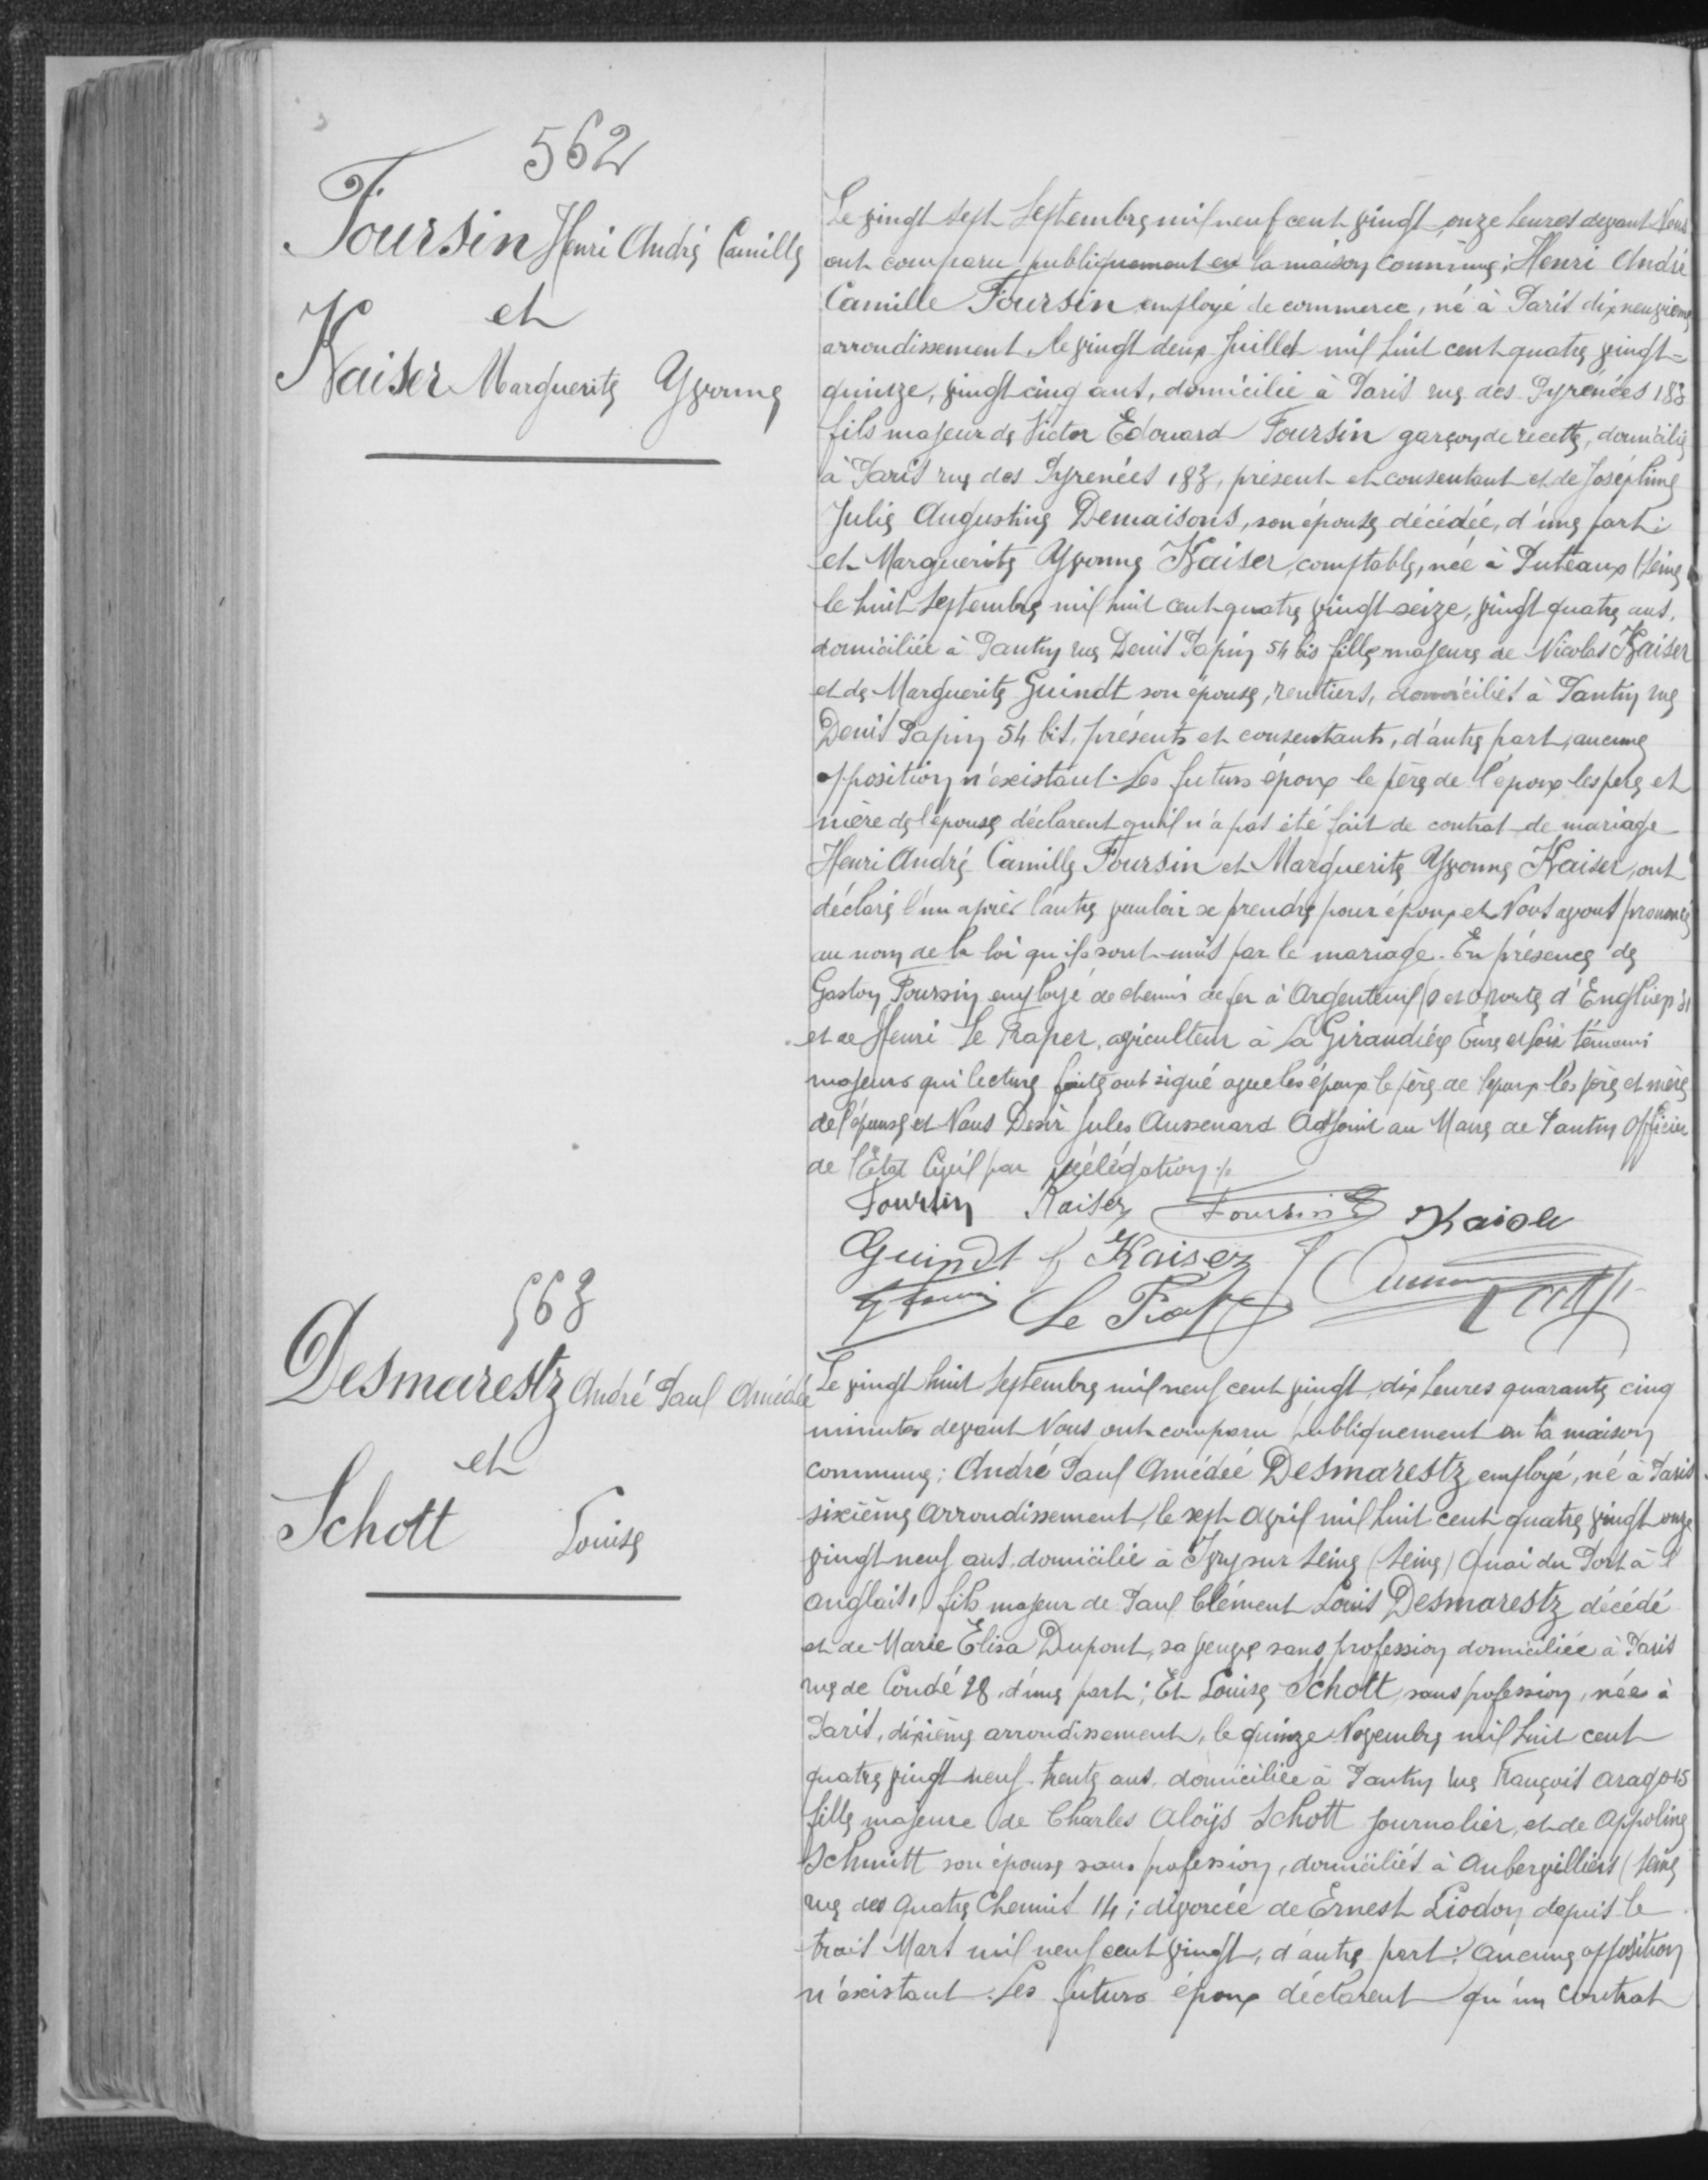562
Foursin Henri André Camille
et
Kaiser Marguerite Yvonne
Le vingt sept Septembre mil neuf cent vingt onze heure devant Nous
ont comparu publiquement en la maison commune; Henri André
Camille Foursin employé de commerce, né à Paris dix neuvième
arrondissement, le vingt deux Juillet mil huit cent quatre vingt-
quinze, vingt cinq ans, domiciliée à Paris rue de Pyrénées 183
fils majeur de Victor Edouard Foursin garçon de recette domicilié
à Paris rue des Pyrenées 183, présent et consentant, et de Joséphine
Julie Augustine Demaisons, son épouse, décédée, d'une part.
et Marguerite Yvonne Kaiser, comptable, née à Puteaux (Seine
le huit Septembre mil huit cent-quatre vingt-seize, vingt quatre ans,
domiciliée à Pantin rue Denis Papin, 54 bis fille majeure de Nicolas Kaiser
et de Marguerite Guindt son épouse, rentiers, domiciliés à Pantin rue
Denis Papin, 54 bis présents et consentants, d'autre part, aucune
opposition n'existant. Les futurs époux le père de l'époux les pere et
mère de l'épouse déclarent qu'il n'a pas été fait de contrat de mariage
Henri André Camille Foursin et Marguerite Yvonne Kaiser, ont
déclaré l'un après l'autre vouloir se prendre pour époux et Nous avons prononcé
au nom de la loi qu'ils sont unis par le mariage. En présence de
Gaston Foursin employé de chemin de fer à Argenteuil (S et O) route d'Englien 31
et de Henri le Frapier, agriculteur à la Giraudière Eure et Loir témoins
majeurs qui lecture faite ont signé avec les époux, le père de l'époux, les père et mère
de l'épouse et Nous Desir Jules Aussenard Adjoit au Maire de Pantin Officier
de l'Etat Civil par délégation ./.
563
Desmarestz André Paul Amédée
et
Schott Louise
Le vingt huit septembre mil neuf cent vingt dix heures quanrante cinq
minutes devant Nous ont comparu publiquement en la maison,
commune: André Paul Amédée Desmarestz, employé, né à Paris
sixième Arrondissement, le sept Avril mil huit cent quatre vingt onze
vingt-neuf ans domicilié à Issy sur Seine (Seine) Quai du Port à l'
Anglais, fils majeur de Paul Clément Louis Desmarestz décédé
et de Marie Elisa Dupont, sa veuve sans profession, domiciliée à Paris
rue de Condé 28, d'une part; Et Louise Schott, sans profession, née à
Paris, dixième arrondissement, le quinze Novembre mil huit cent
quatre vingt neuf, trente ans, domicilié à Pantin rue François Arago 15
fille majeure de Charles Aloys Schott journalier et de Appoline
Schmitt son épouse, sans profession, domiciliés à Aubervilliers (Seine
rue des Quatre Chemins, 14; divorcée de Ernest Liodoy, depuis le
trois Mars mil neuf cent vingt, d'autre part: Aucune opposition
n'existant. Les futurs époux déclarent qu'un contrat
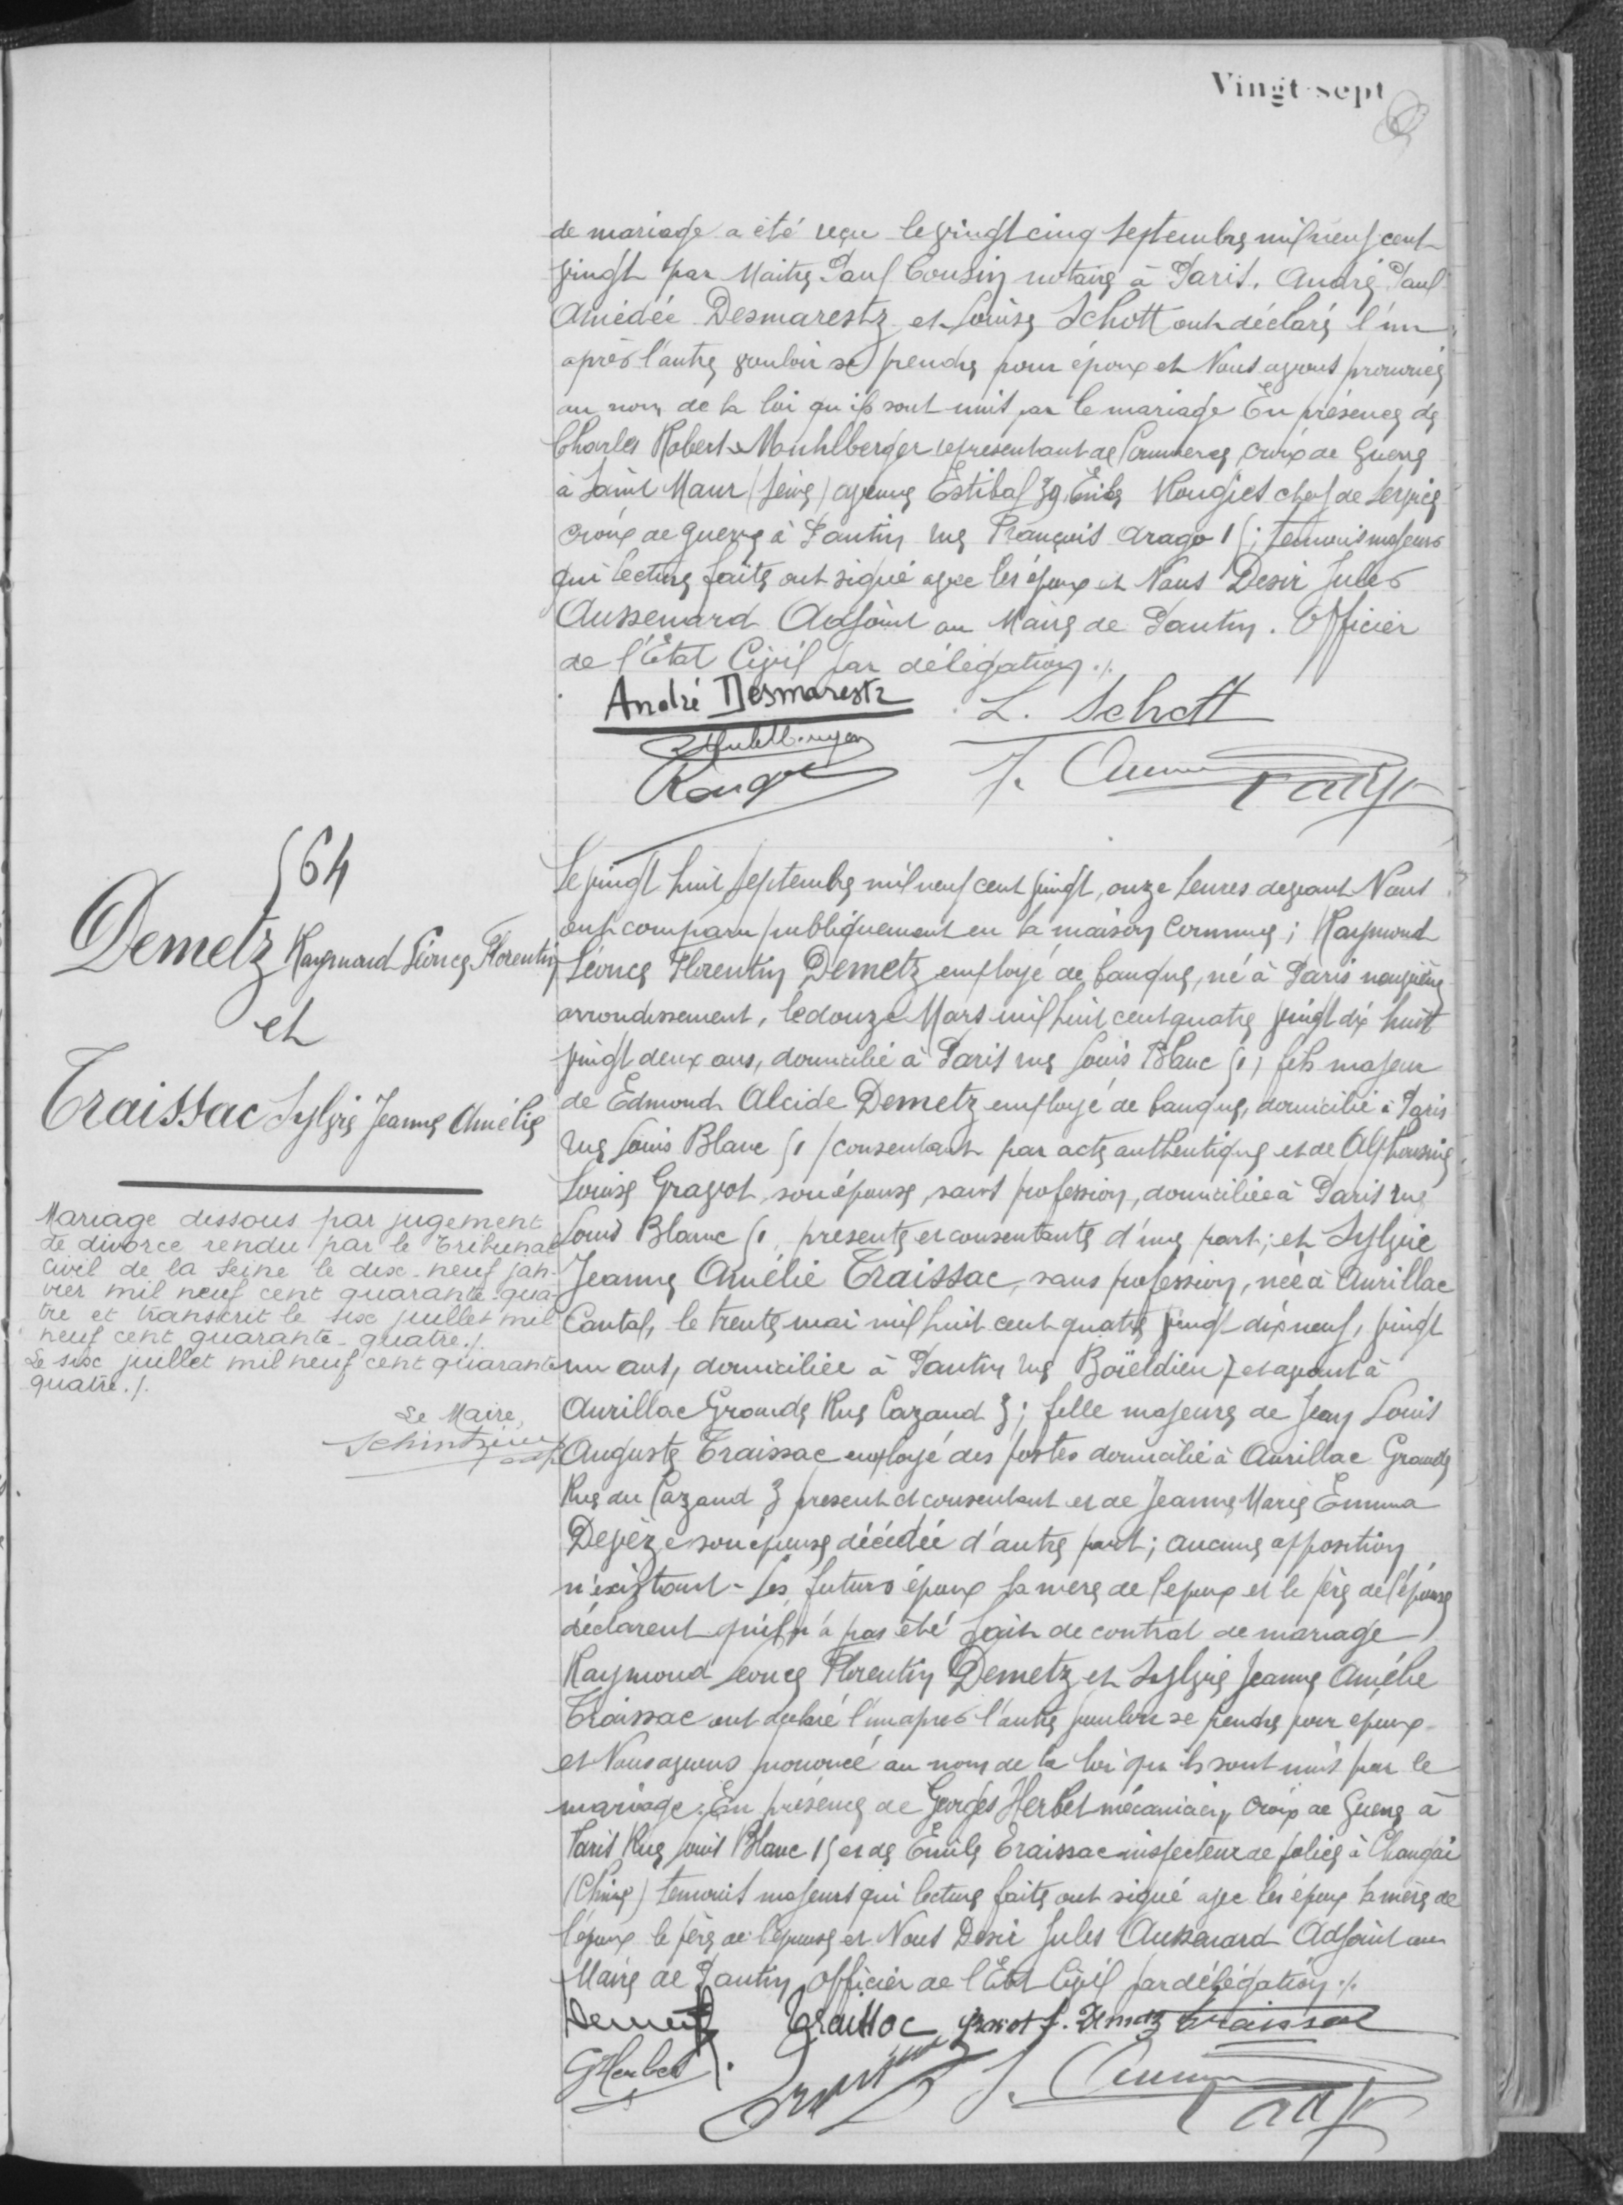de mariage a été reçu le vingt cinq septembre mil neuf cent
vingt par Maitre Paul Cousin notaire à Paris. André Paul
Amédé Desmarestz et Louise Schott ont déclaré, l'un
après l'autre vouloir se prendre pour époux et Nous avons prononcés
au nom de la loi qu'ils sont unis par le mariage En présence de
Charles Robert Muchberger representant de Commerce, croix de guerre
à Saint Maur (Seine) avenue Estibaf 39 Emile Rougies chef de Service
Croix de guerre à Pantin rue François Arago 1; temoins majeurs
qui lecture faite ont signé avec les époux et Nous Desir Jules
Aussevard Adjoint au Maire de Pantin. Officier
de l'Etat Civil par délégation.
564
Demetz Ragnard Léonce Florentin
et
Traissac Sylvie Jeanne Amélie
Le vingt huit septembre mil neuf cent vingt, onze heures devant Nous
ont comparu publiquement en la maison commune; Raymond
Léonce Florentin Demetz employé de banque, né à Paris neuvième
arrondissement, le douze Mars mil huit cent quatre vingt dix huit
vingt deux ans, domicilié à Paris rue Louis Blanc 51, fils majeure
de Edmond Alcide Demetz employé de banque, domicilié à Paris
rue Louis Blave 51/ consentant par acte authentique et de Alphonsine
Louise Gravot, son épouse sans profession, domiciliée à Paris rue
Louis Blanc 51, présents et consentants d'une part; et Sylvie
Jeanne Aurélie Traissac, sans profession, née à Aurillac
Cantal, le trente mai mil huit cent quatre vingt dix neuf, vingt
un ans, domiciliée à Pantin rue Boïeldieu, et avant à
Aurillac Grande Rue Cazaud 3; fille majeure de Jean Louis
Auguste Traissac employé des postes domicilié à Aurillac Grande
Rue du Cazaud 3, present et consentant et de Jeanne Marie Emma
Depèze son epouse décédée d'autre part; Aucune opposition
n'existant-Les futurs époux la mere de lepoux et le père de l'épouse
déclarent qu'il n'a pas été fait de contrat de mariage
Raymond Leviez Florentin Demetz et Sylvie Jeanne Amélie
Traissac ont déclaré l'un apres l'autre vouloir se prendre pour epoux.
et Nous avons prononcé au nom de la loi qu'ils sont unis par le
mariage. En présence de Garces Herbel mécanicien, Croix de Guerre à
Paris Rue Louis Blanc 15 et de Emile Traissac inspecteur de Jolies à Changai
(Chine) transcris majeurs qui lecture faite on signé avec les époux la mère de
l'époux le père de l'époux et Nous Désir Aukenard Jules Adjoint au
Maire de Pantin Officier de l'Etat Civil par délégation./.